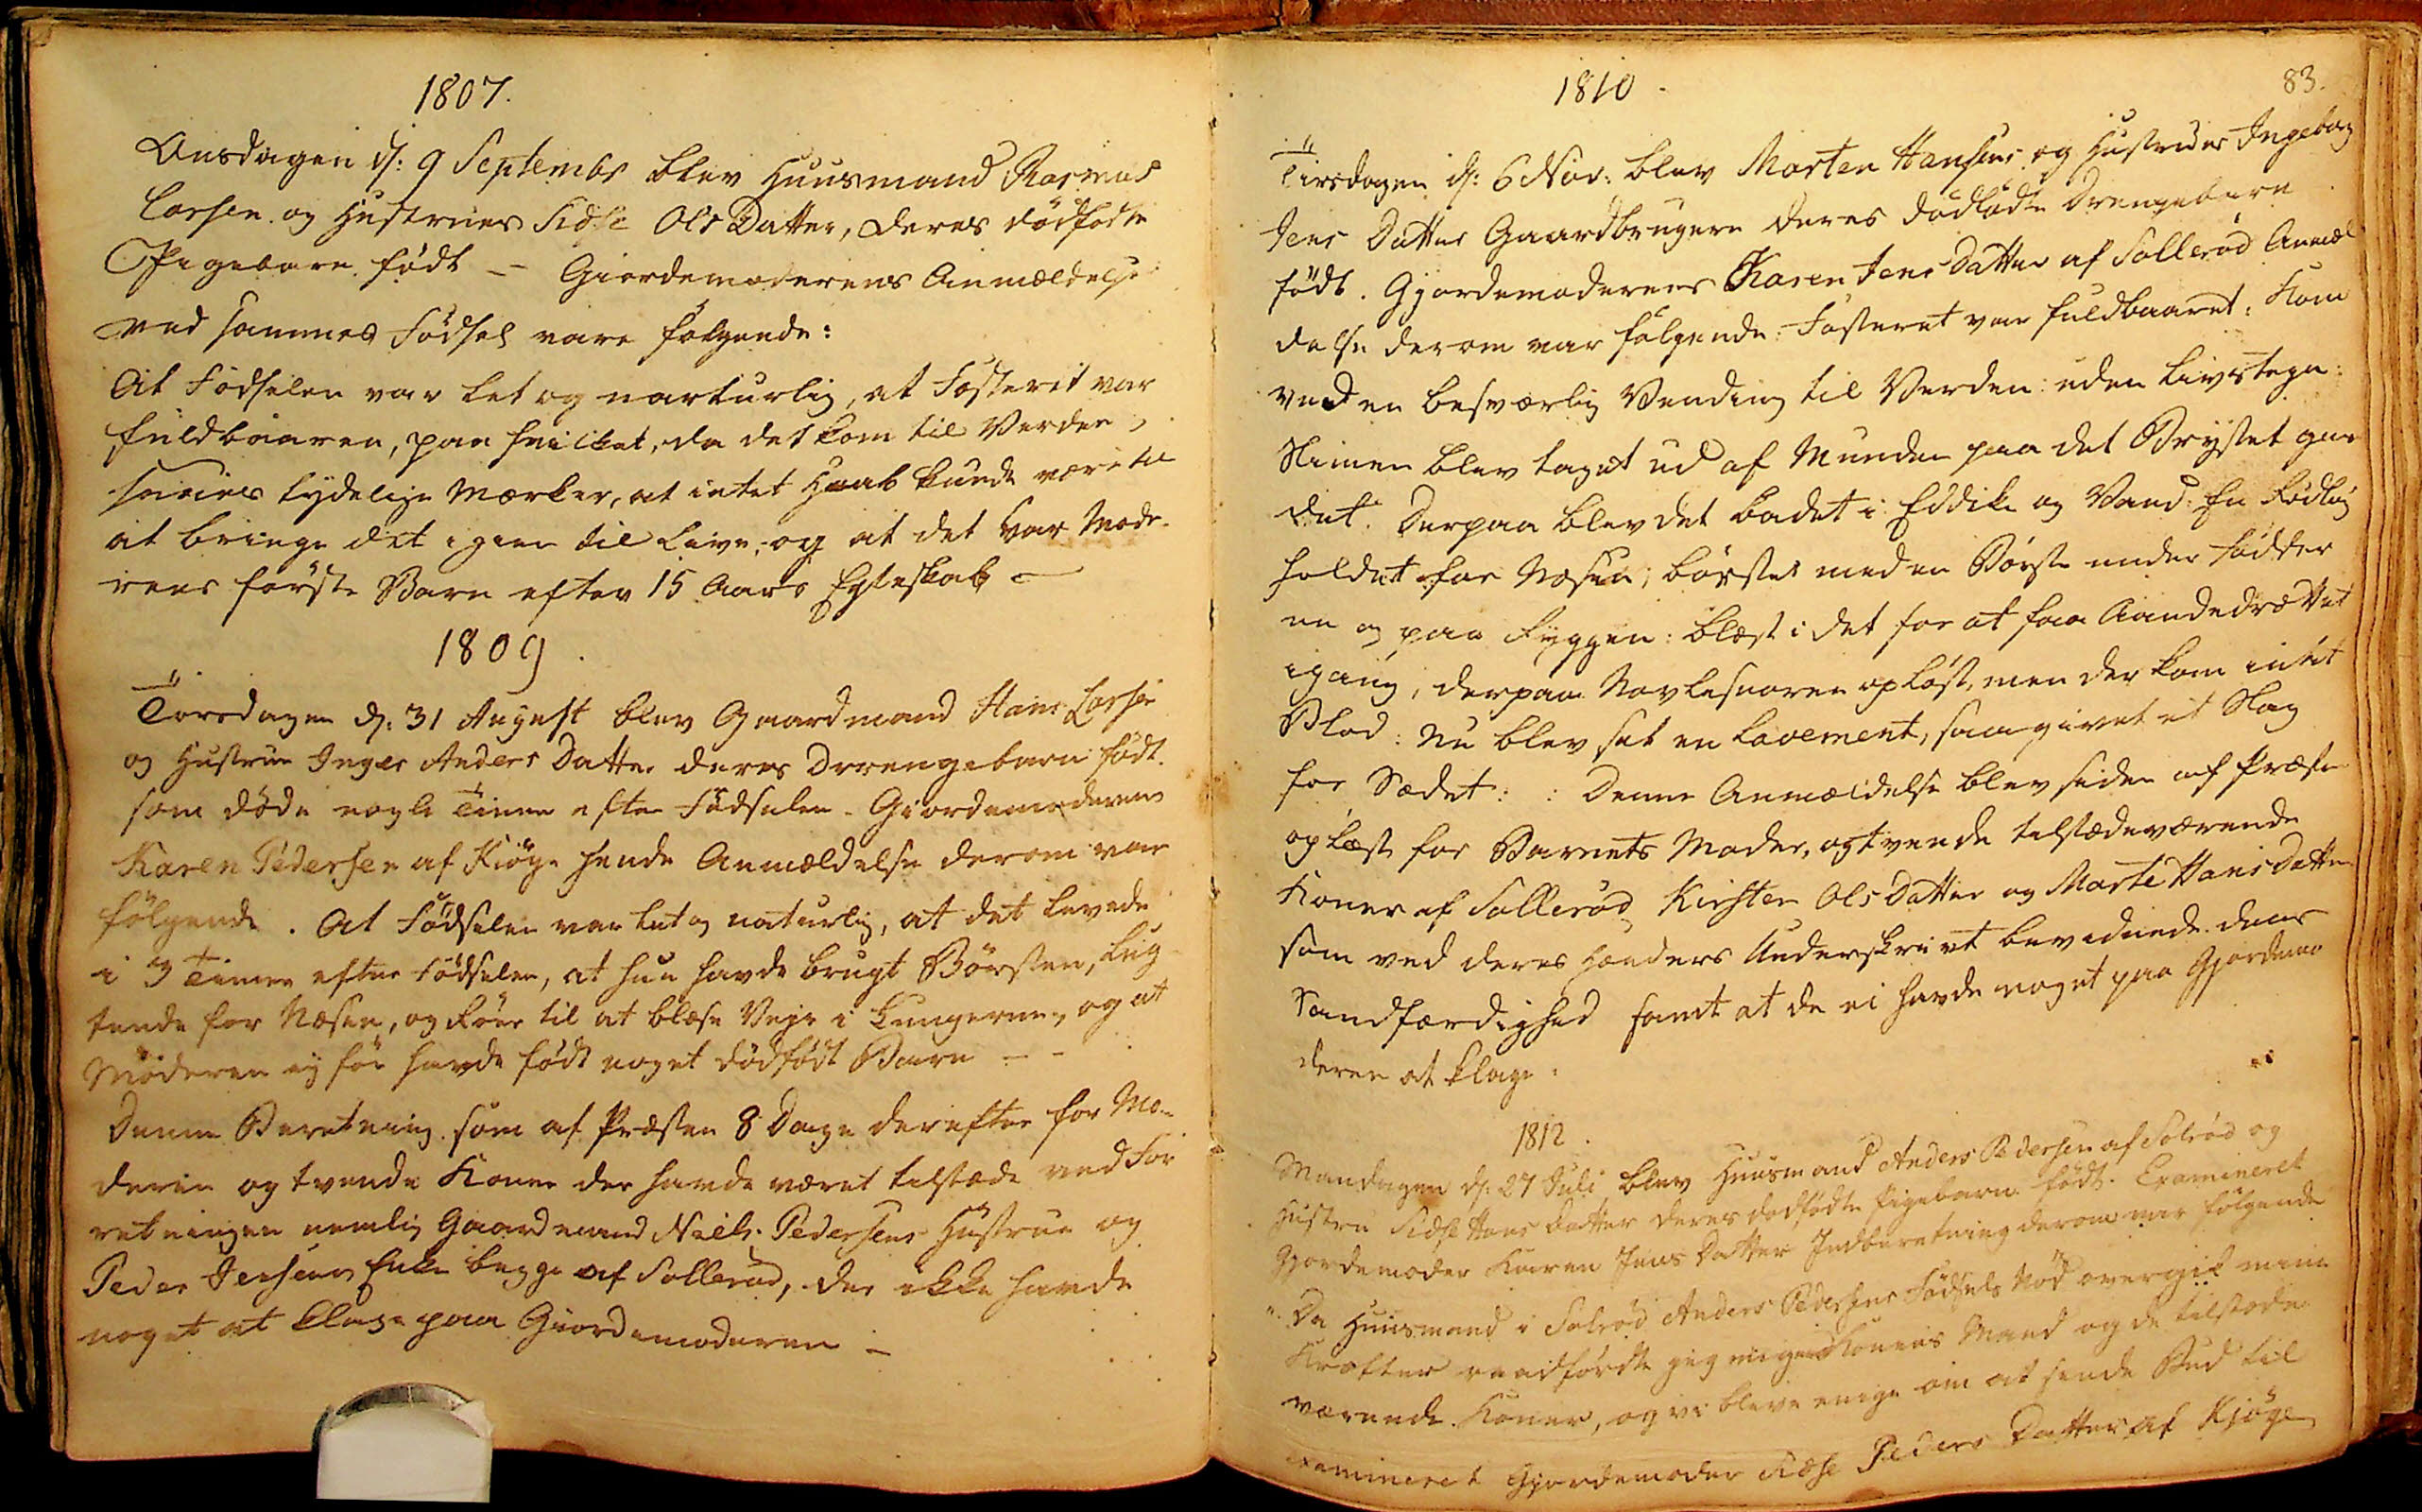1807.
Onsdagen d: 9 Septembr blev Huusmand Rasmus
Larsen og Hustrues Sidse OlsDatter, deres dødfødte
Pigebarn født - - Giordemoderens Anmældelse
ved sammes Fødsel vare følgende:
At Fødselen var let og narturlig, at Fosteret vare
fuldbaaren, paa hvilket, da det kom til Verden,
sanes tydelige Mærker, at intet Havt kunde være til
at bringe det igen til live, og at det var Mode¬
rens første Barn efter 15 Aars Egteskab
1809
Torsdagen d: 31 August blev Gaardmand Hans Larsen
og Hustrue Inger Anders Datter deres Drengebarn født
som døde nogle Timer efter Fødselen. Giordemoderens
Karen Pedersen af Kiøge hendes Anmældelse derom var
følgende. At Fødselen var let og naturlig, at det levede
i 3 Timer efter Fødselen, at hun havde brugt Børsten, lug
tende for Næsen, og Røer til at blæse Vejr i Lungerne, og at
Moderen ej før havde født noget dødfødt Barn
Denne Beretning som af Præsten 8 Dage derefter for Mo¬
deren og tvende Koner der havde været tilstæde ved For
retningen nemlig Gaardmand Niels Pedersens Hustrue og
Peder Jensens Enke begge af Sollerød, der ikke havde
noget at klage paa Giordemoderen
83.
1810.
Tirsdagen d: 6 Nov: blev Morten Hansens og hustrues Ingeborg
Jens Datter Gaardbrugere deres dødfødte Drengebarn
født. Gjordemoderens Karen Jens Datter af Sollerød Anmæl
delse derom var følgende: Fosteret var fuldbaaret: Kom
under besværlig Vending til Verden: uden Livstegn:
Slimen blev taget ud af Munden paa det. Brystet gen##
det. Derpaa blev det badet i Eddike og Vand: En Rødløg
holdet for Næsen; børstet med en Børste under Fødder
ne og paa Ryggen: blæst i det for at faa Aandedrættet
igang, derpaa Navlesnoren opløst, men der kom intet
Blod: nu blev sat en lavement, sam givet et Slag
for Sædet: : Denne Anmældelse blev siden af Præsten
og læst for Barnets Moder, og tvende tilstædeværende
Koner af Sollerød Kirsten Ols Datter og Marte Hans Datter
som ved deres Hænders Underskrivt bevidnede dens
Sandfærdighed samt at de ei havde noget paa Gjordemo
deren at klage.
1812.
Mandagen d: 27 Juli blev Huusmand Anders Pedersen af Solrød og
Hustru Sidse Hans Datter deres dødfødte Pigebarn født. Examineret
Gjordemoder Karen Jens Datter Indberetning derom var følgende
"Da Huusmand i Solrød Anders Pedersens Fødsels Nød overgik mine
Kræfter raadførdte jeg mig med Konens Mand og de tilstæde
værende Koner, og vi blev enige om at sende Bud til
examineret Gjordemoder Sidse Peders Datter af Kjøge